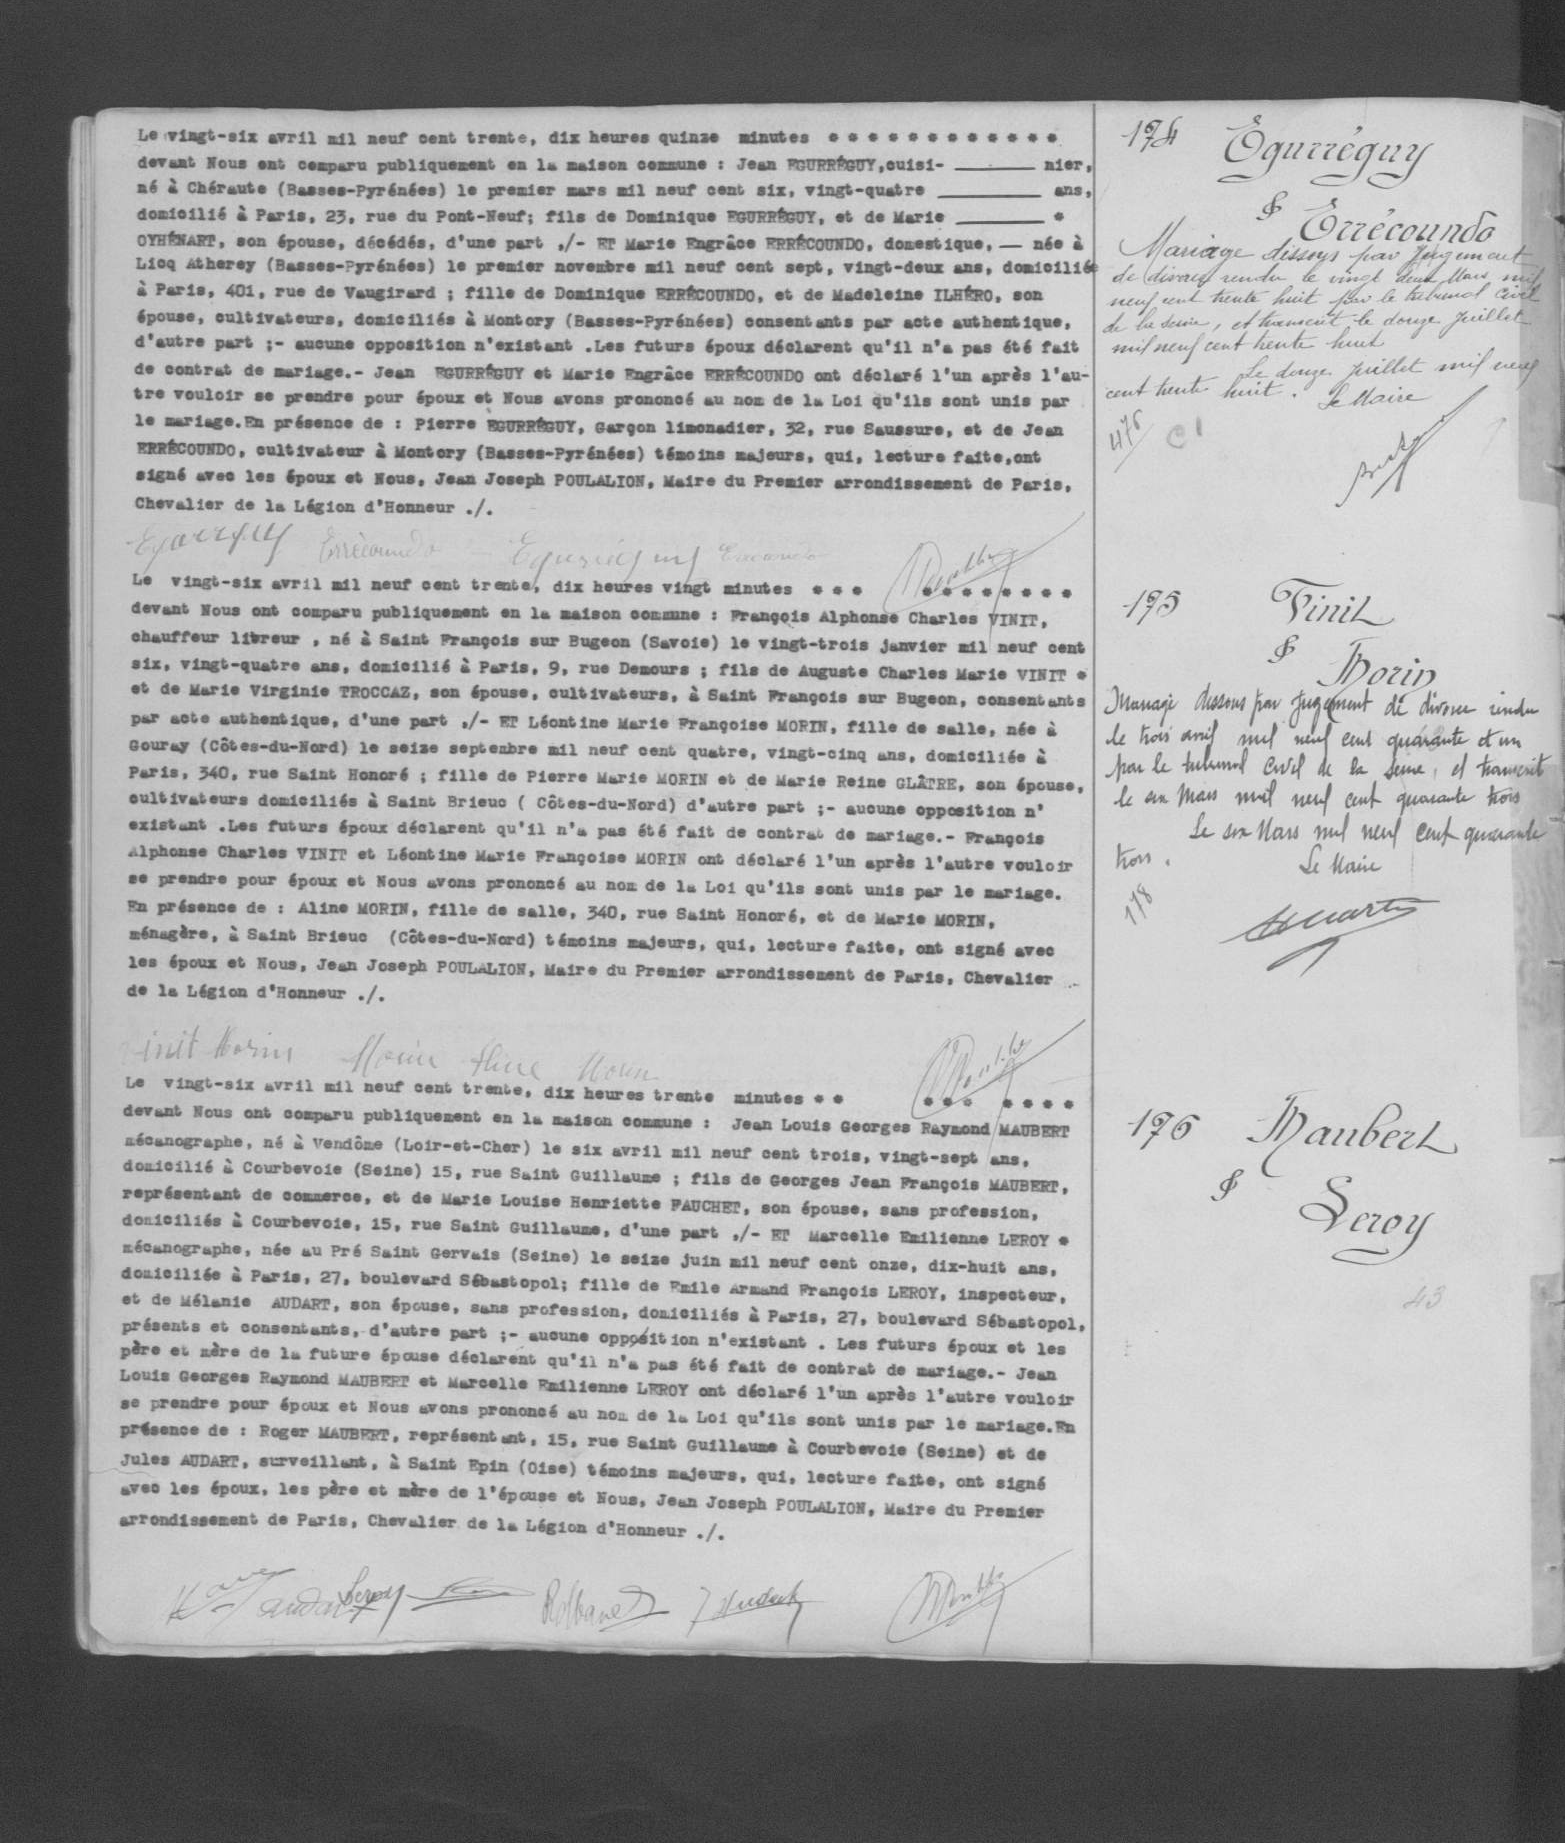Le vingt-six avril mil neuf cent trente, dix heures quinze minutes ****
devant Nous ont comparu publiquement en la maison commune: Jean EGURREGUY, cuisi -nier,
né à Chéraute (Basses-Pyrénées) le premier mars mil neuf cent six, vingt-quatre -ans,
domicilié à Paris, 23, rue du Pont-Neuf; fils de Dominique EGURREGUY, et de Marie -*
OYHENART, son épouse, décédés, d'une part ,/-ET Marie Engrâce ERRECOUDO, domestique, -née à
Licq Atherey (Basses-Pyrénées) le premier novembre mil neuf cent sept, vingt-deux ans, domiciliée
à Paris, 401, rue de Vaugirard; fille de Dominique ERRECOUNDO, et de Madeleine ILHERO, son
épouse, cultivateurs, domiciliés à Montory (Basses-Pyrénées) consentants par acte authentique,
d'autre part ;-aucune opposition n'existant. Les futurs époux déclarent qu'il n'a pas été fait
de contrat de mariage .-Jean EGURREGUY et Marie Engrâce ERRECOUNDO ont déclaré l'un après l'au-
tre vouloir se prendre pour époux et Nous avons prononcé au nom de la Loi qu'ils sont unis par
le mariage. En présence de: Pierre EGURREGUY, Garçon limonadier, 32, rue Saussure, et de Jean
ERRECOUNDO, cultivateur à Montory (Basses-Pyrénées) témoins majeurs, qui, lecture faite, ont
signé avec les époux et Nous, Jean Joseph POULALION, Maire du Premier arrondissement de Paris,
Chevalier de la Légion d'Honneur ./.
Le vingt-six avril mil neuf cent trente, dix heures vingt minutes ****
devant Nous ont comparu publiquement en la maison commune: François Alphonse Charles VINIT,
Chauffeur livreur, né à Saint François sur Bugeon (Savoie) le vingt-trois janvier mil neuf cent
six, vingt-quatre ans, domicilié à Paris, 9, rue Demours; fils de Auguste Charles Marie VINIT *
et de Marie Virginie TROCCAZ, son épouse, cultivateurs, à Saint François sur Bugeon, consentants
par acte authentique, d'une part ,/-ET Léontine Marie Françoise MORIN, fille de salle, née à
Gouray (Côtes-du-Nord) le seize septembre mil neuf cent quatre, vingt-cinq ans, domiciliée à
Paris, 340, rue Saint Honoré; fille de Pierre Marie MORIN et de Marie Reine GLATRE, son épouse,
cultivateurs domiciliés à Saint Brieue (Côtes-du-Nord) d'autre part ;-aucune opposition n'
existant. Les futurs époux déclarent qu'il n'a pas été fait de contrat de mariage .-François
Alphonse Charles VINIT et Léontine Marie Françoise MORIN ont déclaré l'un après l'autre vouloir
se prendre pour époux et Nous avons prononcé au nom de la Loi qu'ils sont unis par le mariage.
En présence de: Aline MORIN, fille de salle, 340, rue Saint Honoré, et de Marie MORIN,
ménagère, à Saint Brieue (Côtes-du-Nord) témoins majeurs, qui, lecture faite, ont signé avec
les époux et Nous, Jean Joseph POULALION, Maire du Premier arrondissement de Paris, Chevalier
de la Légion d'Honneur ./.
Le vingt-six avril mil neuf cent trente, dix heures trente minutes ****
devant Nous ont comparu publiquement en la maison commune: Jean Louis Georges Raymond MAUBERT
mécanographe, né à Vendôme (Loir-et-Cher) le six avril mil neuf cent trois, vingt-sept ans,
domicilié à Courbevoie (Seine) 15, rue Saint Guillaume; fils de Georges Jean François MAUBERT,
répresentant de commerce, et de Marie Louise Henriette FAUCHET, son épouse, sans profession,
domiciliés à Courbevoie, 15, rue Saint Guillaume, d'une part ,/-ET Marcelle Emilienne LEROY *
mécanographe, née au Pré Saint Gervais (Seine) le seize juin mil neuf cent onze, dix-huit ans,
domiciliée à Paris, 27, boulevard Sébastopol; fille de Emile Armand François LEROY, inspecteur,
et de Mélanie AUDART, son épouse, sans profession, domiciliés à Paris, 27 boulevard Sébastopol,
présents et consentants, d'autre part ;-aucune opposition n'existant. Les futurs époux et les
père et mère de la future épouse déclarent qu'il n'a pas été fait de contrat de mariage .-Jean
Louis Georges Raymond MAUBERT et Marcelle Emilienne LEROY ont déclaré l'un après l'autre vouloir
se prendre pour époux et Nous avons prononcé au nom de la Loi qu'ils sont unis par le mariage. En
présence de: Roger MAUBERT, représentant, 15, rue Saint Guillaume à Courbevoie (Seine) et de
Jules AUDART, surveillant, à Saint Epin (Oise) témoins majeurs, qui, lecture faite, ont signé
avec les époux, les père et mère de l'épouse et Nous, Jean Joseph POULALION, Maire du Premier
arrondissement de Paris, Chevalier de la Légion d'Honneur ./.
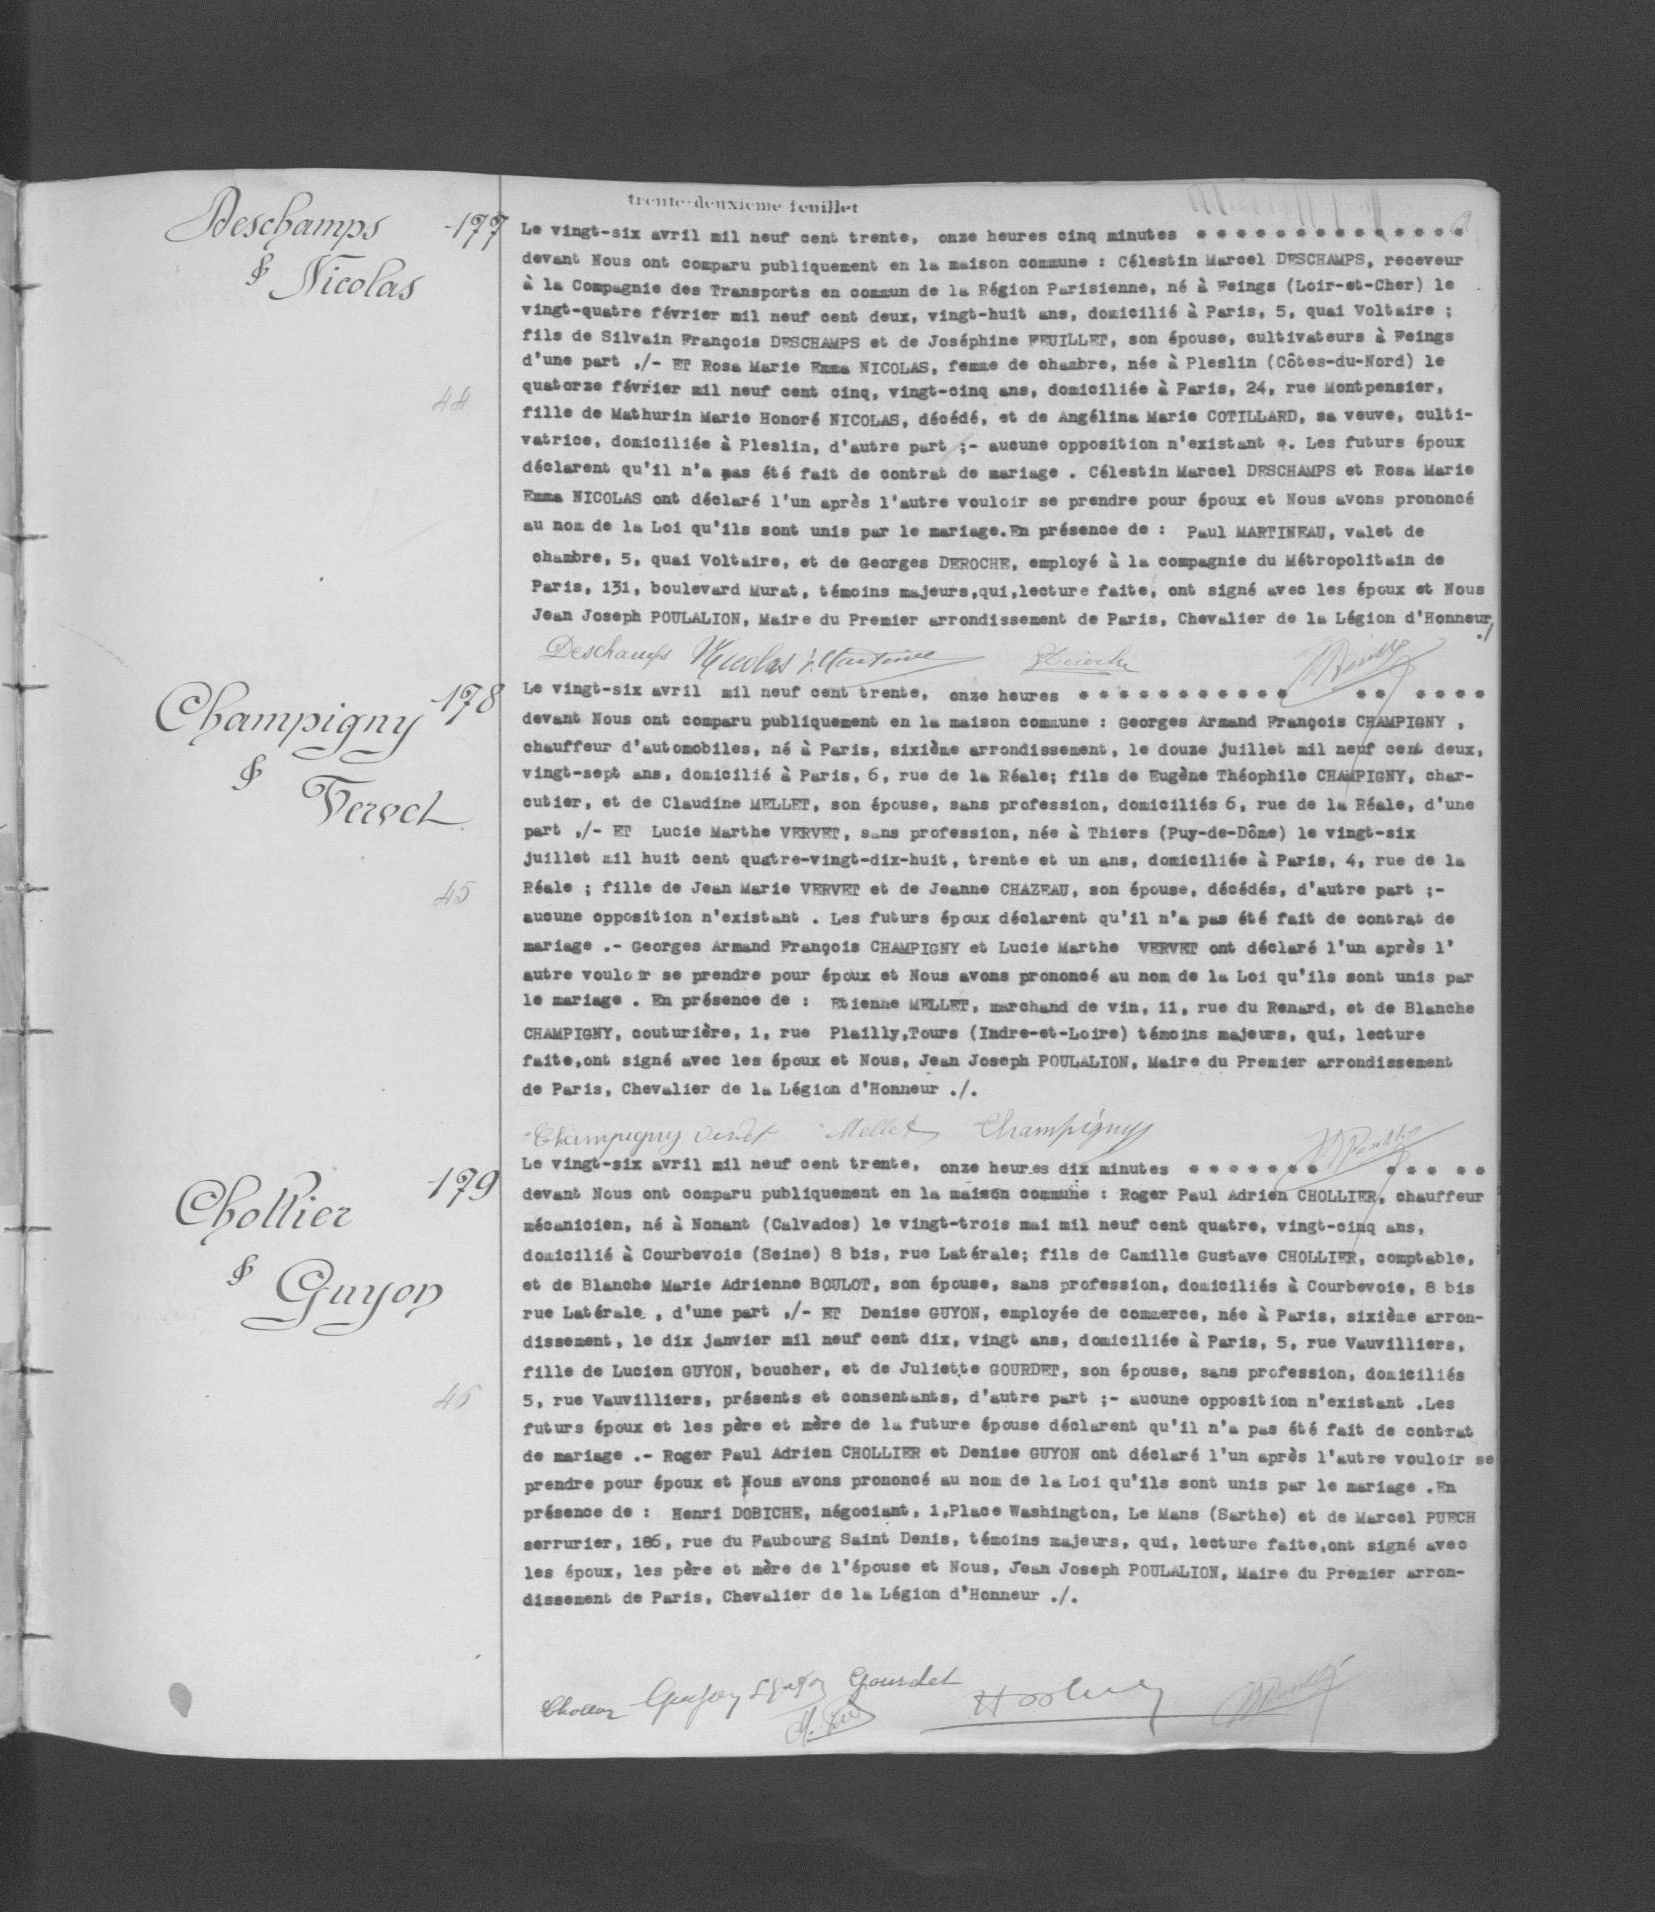Le vingt-six avril mil neuf cent trente, onze heures cinq minutes ****
devant Nous ont comparu publiquement en la maison commune: Célestin Marcel DESCHAMPS, receveur
à la Compagnie des Transports en commun de la Région Parisienne, né à Feings (Loir-et-Cher) le
vingt-quatre février mil neuf cent deux, vingt-huit ans, domicilié à Paris, 5, quai Voltaire;
fils de Silvain François DESCHAMPS et de Joséphine FEUILLET, son épouse, cultivateurs à Feings
d'une part ,/-ET Rosa Marie Emma NICOLAS, femme de chambre, née à Pleslin (Côtes-du-Nord) le
quatorze février mil neuf cent cinq, vingt-cinq ans, domiciliée à Paris, 24, rue Montpensier,
fille de Mathurin Marie Honoré NICOLAS, décédé, et de Angélina Marie COTILLARD, sa veuve, culti-
vatrice, domiciliée à Pleslin, d'autre part ;-aucune opposition n'existant *. Les futurs époux
déclarent qu'il n'a pas été fait de contrat de mariage. Célestin Marcel DESCHAMPS et Rosa Marie
Emma NICOLAS ont déclaré l'un après l'autre vouloir se prendre pour époux et Nous avons prononcé
au nom de la Loi qu'ils sont unis par le mariage. En présence de: Paul MARTINEAU, valet de
chambre, 5, quai Voltaire, et de Georges DEROCHE, employé à la compagnie du Métropolitain de
Paris, 131, boulevard Murat, témoins majeurs, qui, lecture faite, ont signé avec les époux et Nous
Jean Joseph POULALION, Maire du Premier arrondissement de Paris, Chevalier de la Légion d'Honneur ./
Le vingt-six avril mil neuf cent trente, onze heures ****
devant Nous ont comparu publiquement en la maison commune: Georges Armand François CHAMPIGNY,
chauffeur d'automobiles, né à Paris, sixième arrondissement, le douze juillet mil neuf cent deux,
vingt-sept ans, domicilié à Paris, 6, rue de la Réale; fils de Eugène Théophile CHAMPIGNY, char-
cutier, et de Claudine MELLET, son épouse, sans profession, domiciliés 6, rue de la Réale, d'une
part ,/-ET Lucie Marthe VERVET, sans profession, née à Thiers (Puy-de-Dôme) le vingt-six
juillet mil neuf cent quatre-vingt-dix-huit, trente et un ans, domiciliée à Paris, 4, rue de la
Réale; fille de Jean Marie VERVET et de Jeanne CHAZEAU, son épouse, décédés, d'autre part ;-
aucune opposition n'existant. Les futurs époux déclarent qu'il n'a pas été fait de contrat de
mariage .-Georges Armand François CHAMPIGNY et Lucie Marthe VERVET ont déclaré l'un après l'
autre vouloir se prendre pour époux et Nous avons prononcé au nom de la Loi qu'ils sont unis par
le mariage. En présence de: Etienne MELLET, marchand de vin, 11, rue du Renard, et de Blanche
CHAMPIGNY, couturière, 1, rue Plailly, Tours (Indre-et-Loire) témoins majeurs, qui, lecture
faite, ont signé avec les époux et Nous, Jean Joseph POULALION, Maire du Premier arrondissement
de Paris, Chevalier de la Légion d'Honneur ./.
Le vingt-six avril mil neuf cent trente, onze heures dix minutes ****
devant Nous ont comparu publiquement en la maison commune: Roger Paul Adrien CHOLLIER, chauffeur
mécanicien, né à Nonant (Calvados) le vingt-trois mai mil neuf cent quatre, vingt-cinq ans,
domicilié à Courbevoie (Seine) 8 bis, rue Latérale; fils de Camille Gustave CHOLLIER, comptable,
et de Blanche Marie Adrienne BOULOT, son épouse, sans profession, domiciliés à Courbevoie, 8 bis
rue Latérale, d'une part ,/-ET Denise GUYON, employée de commerce, née à Paris, sixième arron-
dissement, le dix janvier mil neuf cent dix, vingt ans, domiciliée à Paris, 5, rue Vauvilliers,
fille de Lucien GUYON, boucher, et de Juliette GOURDET, son épouse, sans profession, domiciliés
5, rue Vauvilliers, présents et consentants, d'autre part ;-aucune opposition n'existant. Les
futurs époux et les père et mère de la future épouse déclarent qu'il n'a pas été fait de contrat
de mariage .-Roger Paul Adrien CHOLLIER et Denise GUYON ont déclaré l'un après l'autre vouloir se
prendre pour époux et Nous avons prononcé au nom de la Loi qu'ils sont unis par le mariage. En
présence de: Henri DOBICHE négociant, 1, Place Washington, Le Mans (Sarthe) et de Marcel PUECH
serrurier, 186, rue du Faubourg Saint Denis, témoins majeurs, qui, lecture faite, ont signé avc
les époux, les père et mère de l'épouse et Nous, Jean Joseph POULALION, Maire du Premier arron-
dissement de Paris. Chevalier de la Légion d'Honneur ./.